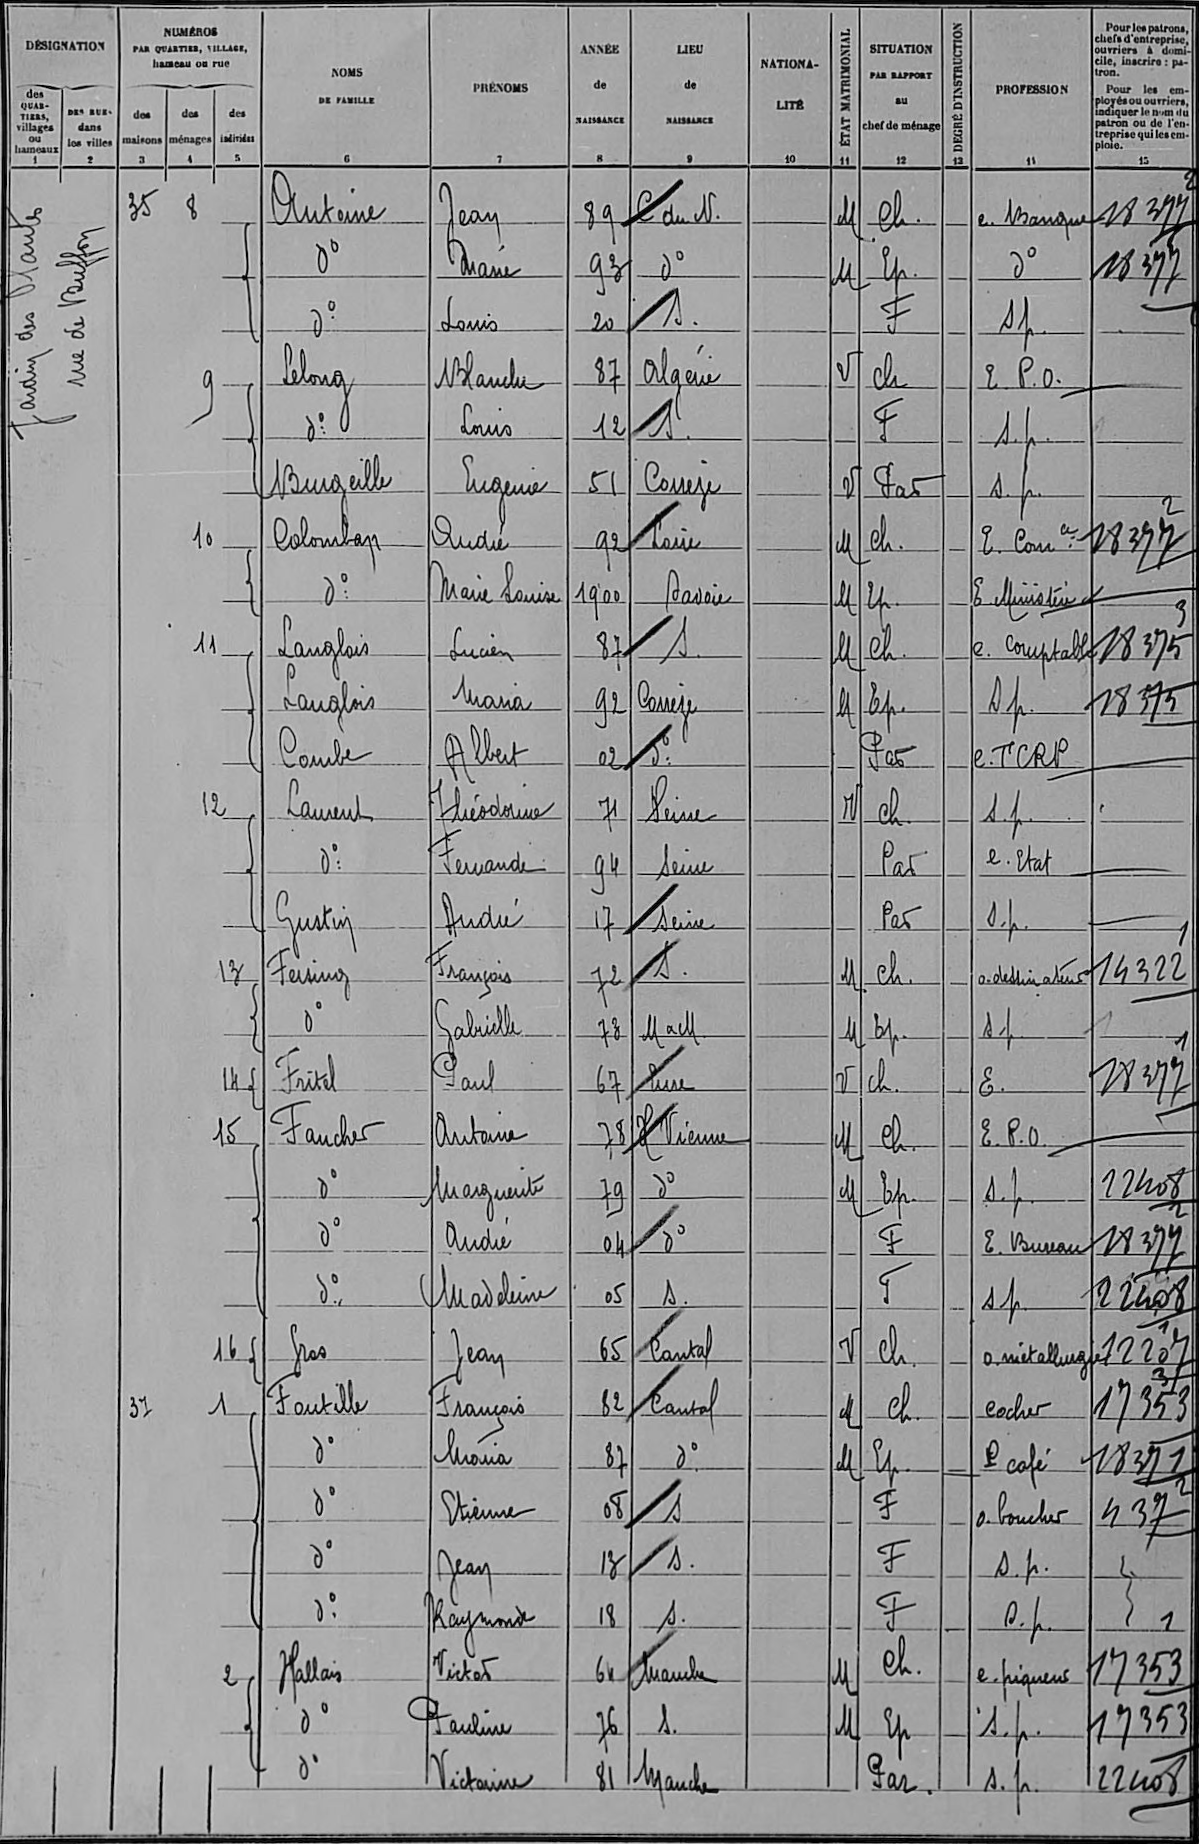Antoine/Jean/89/C du N /¤/M/Ch /¤/e Banque/18377
d°/Marie/93/d°/¤/M/Ep /¤/d°/18377
d°/Louis/20/S /¤/¤/F/¤/Sp/¤
Lelong/Blancge/87/Algérie/¤/V/ch/¤/E P O /¤
d°/Louis/12/S /¤/¤/F/¤/S p /¤
Burgeille/Eugénie/51/Corrèze/¤/V/Par/¤/S p /¤
Colombap/André/92/Loire/¤/M/ch /¤/E Comce/18377
d°/Marie Louise/1900/Savoie/¤/M/Ep /¤/E Ministère/¤
Langlois/Lucien/87/S /¤/M/Ch /¤/e comptable/18375
Langlois/Maria/92/Corrèze/¤/M/Ep /¤/S p /18375
Combe/Albert/02/d°/¤/¤/par/¤/e TCRP/¤
Laurent/Théodorine/71/Seine/¤/V/Ch /¤/S p /¤
d°/Fernande/94/seine/¤/¤/Par/¤/e Etat/¤
Gustin/André/17/Seine/¤/¤/Par/¤/S p /¤
Fersing/François/72/S /¤/M/ch /¤/o dessinateur/14322
d°/Gabrielle/73/M &M/¤/M/Ep /¤/S p/¤
Fritel/Paul/67/Eure/¤/V/ch/¤/E /18377
Faucher/Antoine/78/H Vienne/¤/M/Ch /¤/E P P /¤
d°/Marguerite/79/d°/¤/M/Ep /¤/S p /22408
d°/André/04/d°/¤/¤/F/¤/E Bureau/18377
d°/Madelien/05/S /¤/¤/F/¤/S p/22408
Gros/Jean/65/Cantal/¤/V/Ch /¤/o métallurgie/12207
Foutille/François/82/Cantal/¤/M/Ch /¤/cocher/17353
d°/Maria/87/d°/¤/M/Ep /¤/P café/18371
d°/Etienne/08/S/¤/¤/F/¤/o boucher/437
d°/Jean/13/S /¤/¤/F/¤/S p /¤
d°/Raymonde/18/S /¤/¤/F/¤/S p /¤
Hallais/Victor/64/Manche/¤/M/Ch /¤/e piqueur/17353
d°/Pauline/76/S /¤/M/Ep/¤/S p /17353
d°/Victorine/81/Manche/¤/¤/Par /¤/S p /22408
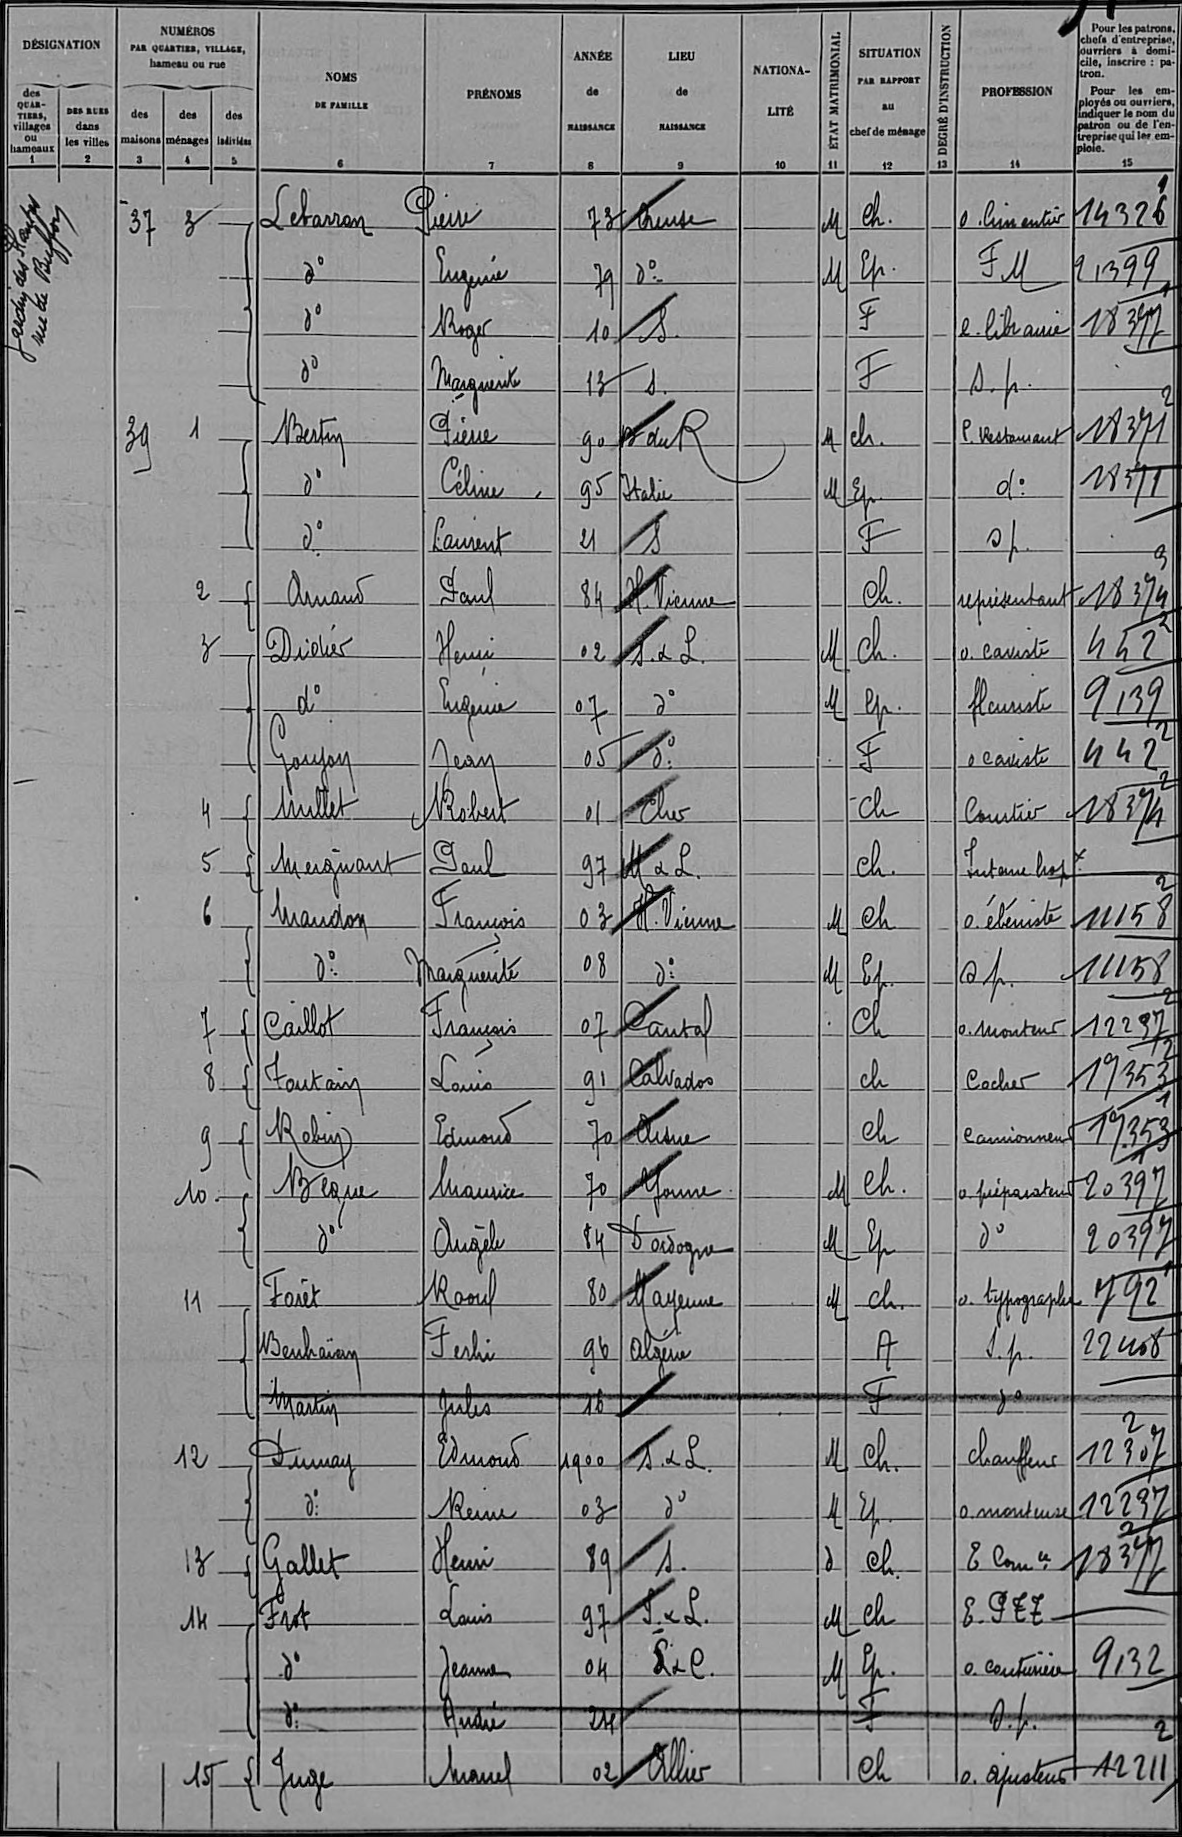Lebarron/Pierre/73/Creuse/¤/M/Ch /¤/o Cimentier/14326
d°/Eugénie/79/d°/¤/M/Ep /¤/F M/21399
d°/Roger/10/S /¤/¤/F/¤/e libraire/18377
d°/Marguerite/13/S /¤/¤/F/¤/S p /¤
Bertin/Pierre/90/B du R/¤/M/ch /¤/P restaurant/18371
d°/Céline/95/Italie/¤/M/Ep /¤/d°/18371
d°/Laurent/21/S/¤/¤/F/¤/Sp/¤
Armand/Paul/84/H VIenne/¤/¤/Ch /¤/représentant/18374
Didier/Henri/02/S & L /¤/M/Ch /¤/o caviste/442
d°/Eugénie/07/d°/¤/M/Ep /¤/fleuriste/9139
Goujon/jean/05/d°/¤/¤/F/¤/o caviste/442
Mullet/Robert/01/Cher/¤/¤/Ch/¤/Courtier/18374
Merginant/Paul/97/M & L /¤/¤/ch /¤/Interne hop/¤
Maudon/François/03/H Vienne/¤/M/Ch/¤/o ébéniste/11158
d°/Marguerite/08/d°/¤/M/Ep /¤/Sp/11158
Caillot/François/07/Cantal/¤/¤/Ch/¤/o monteur/12237
Toutain/louis/91/Calvados/¤/¤/Ch/¤/Cocher/17353
Robin/Edmond/70/Aisne/¤/¤/Ch/¤/camionneur/17353
Beque/Maurice/70/Yonne/¤/M/Ch /¤/o préparateur/20397
d°/Angèle/84/Dordogne/¤/M/Ep/¤/d°/20397
Forêt/Raoul/80/Mayenne/¤/M/ch /¤/o typographe/792
Benhaïm/Ferhi/96/Algérie/¤/¤/A/¤/S p /22408
MArtin/Jules/16/¤/¤/¤/F/¤/d°/¤
Dumay/Edmond/1900/S & L /¤/M/Ch /¤/chauffeur/12307
d°/Reine/03/d°/¤/M/Ep /¤/o monteuse/12237
Gallet/Henri/89/S /¤/d/Ch /¤/E Comce/18377
Frot/Louis/97/I & L/¤/M/ch/¤/E PTT/¤
d°/Jeanne/04/L & C/¤/M/Ep /¤/o couturière/9132
d°/André/24/¤/¤/¤/F/¤/S p /¤
Juge/Marcel/02/Allier/¤/¤/Ch/¤/o ajusteur/12211